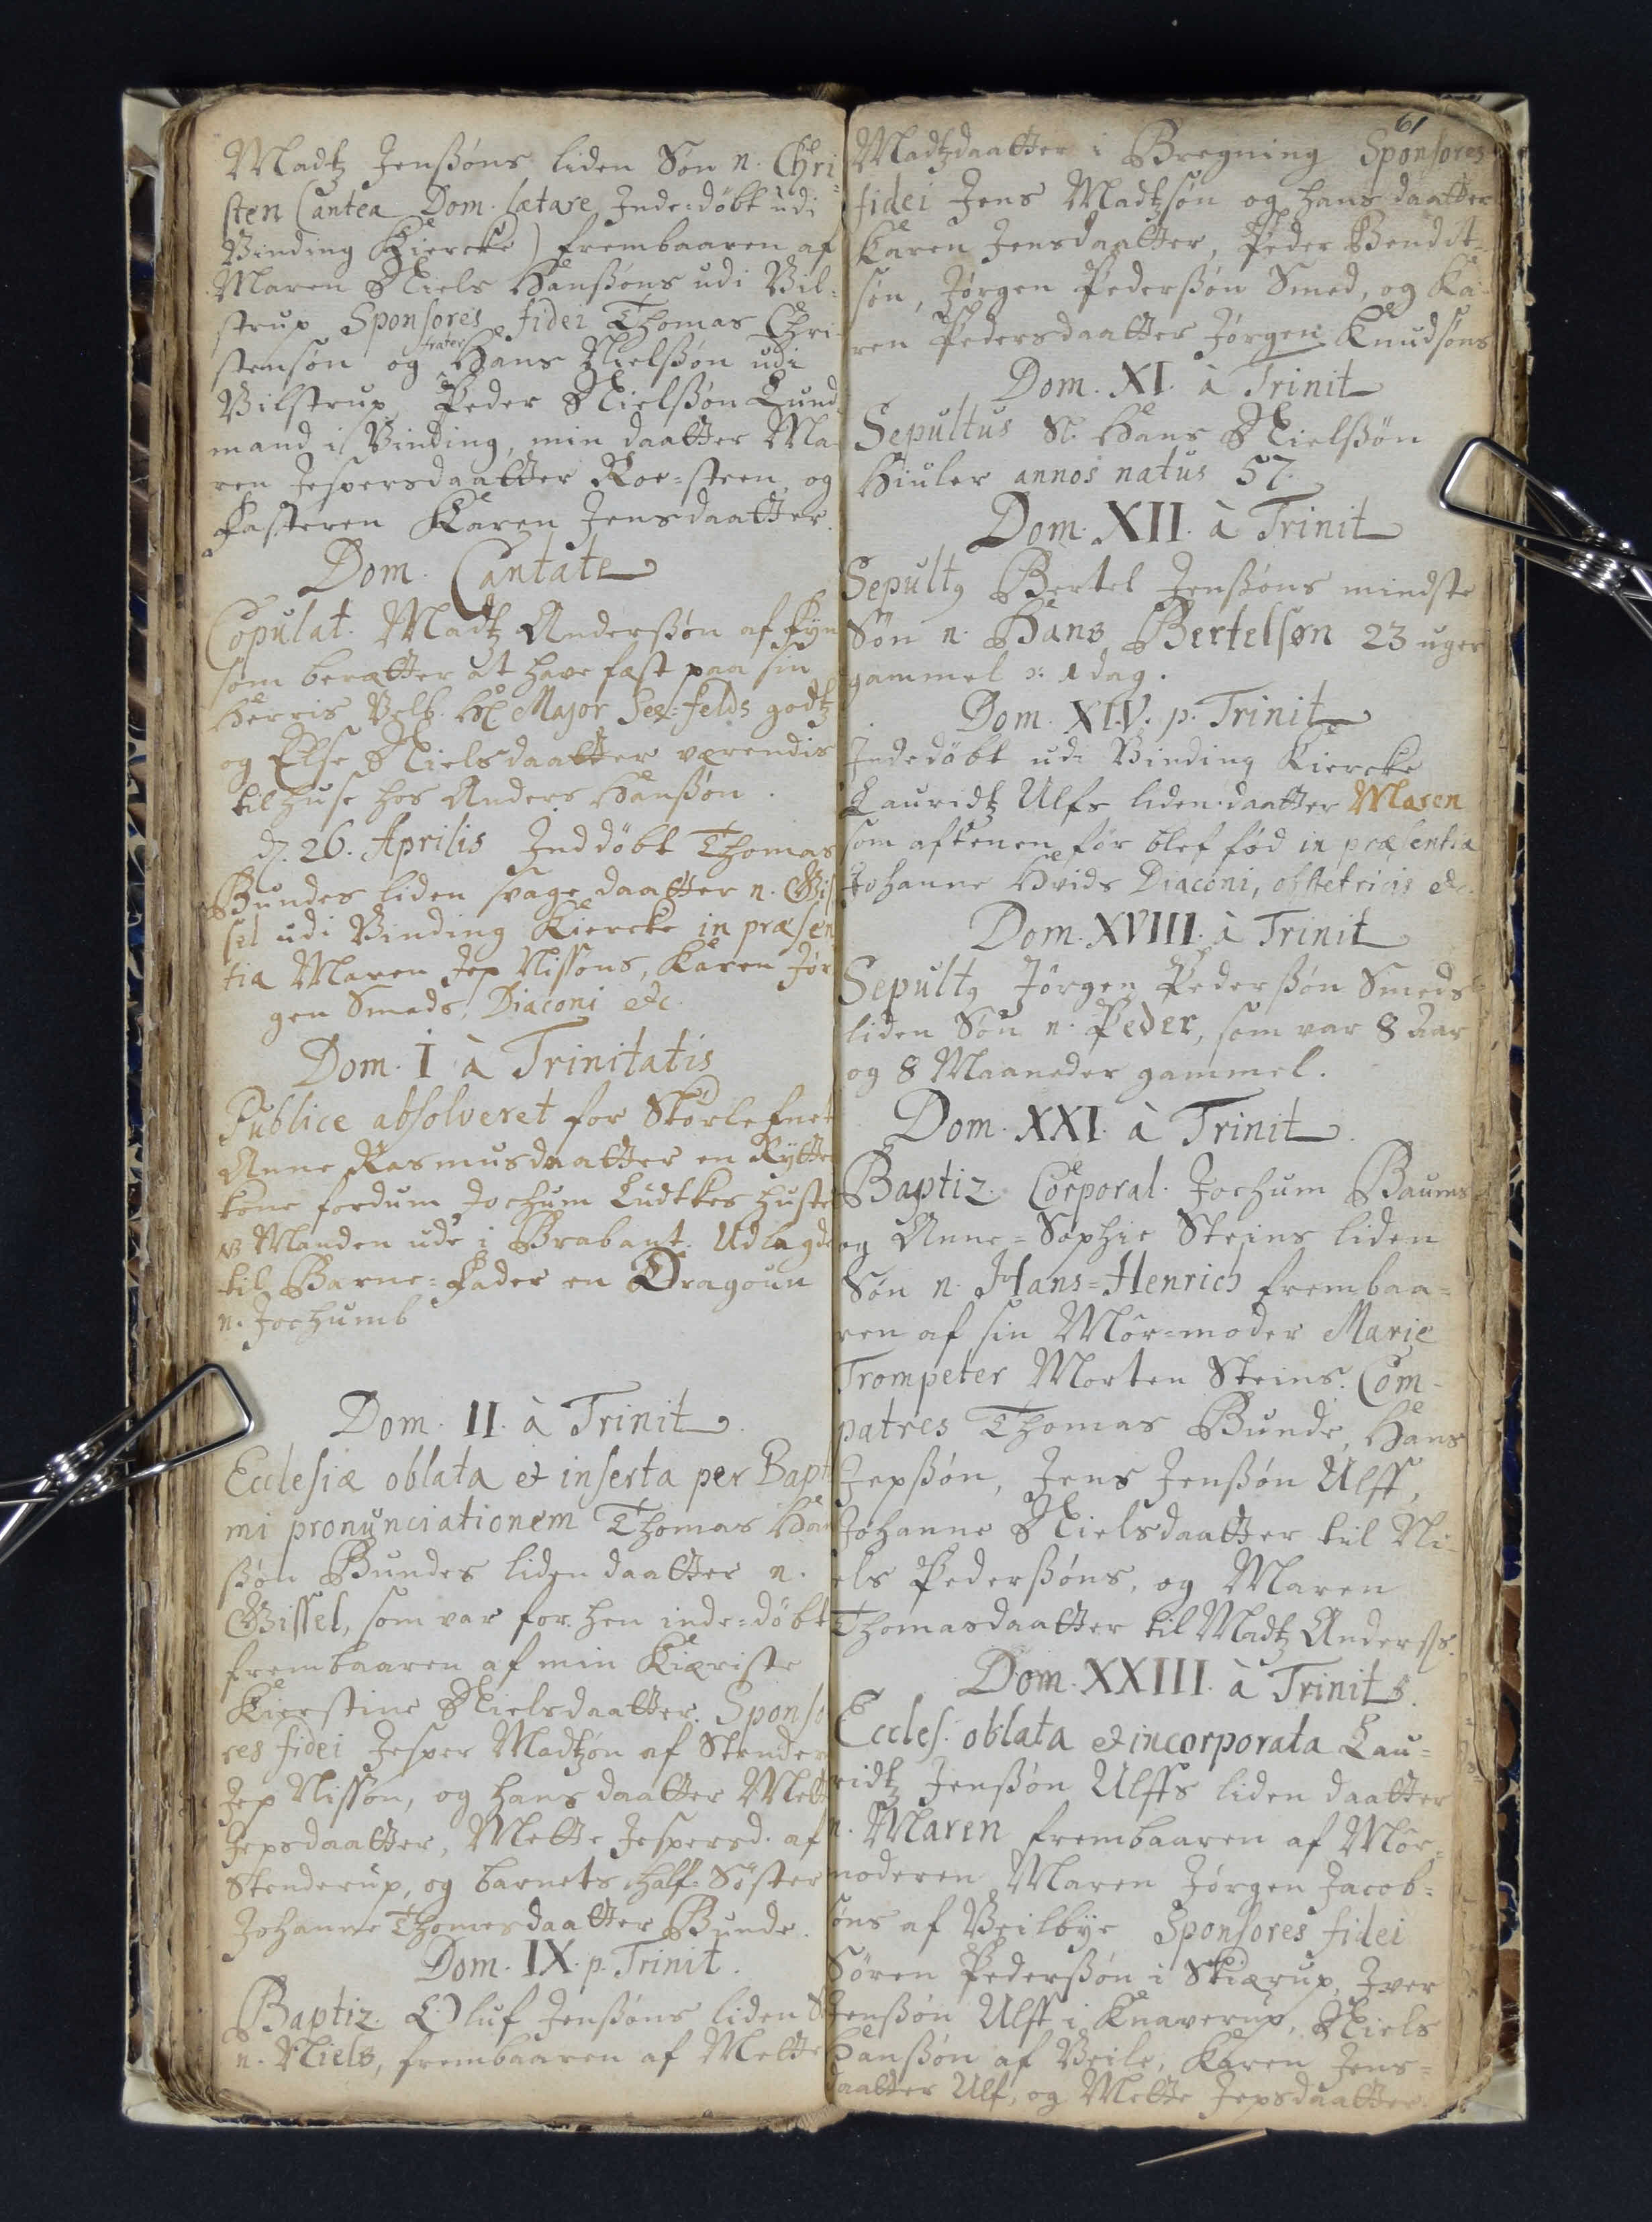Madtz Jensøns liden Søn n. Chri
sten (antea Dom. Lætare Inde=døbt udi
Vinding Kiercke) frembaaren af
Maren Niels Hanssøns udi Vil¬
strup Sponsores fidei Thomas Chri
frater##
stensøn og Hans Nielssøn udi
Vilstrup. Peder Nielssøn Lund
mand i Vinding, min daatter Ma
ren Jespersdaatter Roe=steen, og
Fasteren Karen Jensdaatter
Dom. Cantate
Copult. Madtz Anderssøn af Fyn
som berætter at have fæst paa sin 
Herris Velb. Hl. Major See=felds godtz
og Else Nielsdaatter værendis
til huse hos Anders Hanssøns
d. 26. Aprilis Inddøbt Thomas
Bundes liden svage daatter n. Gis
sel udi Vinding Kiercke in præsen
tia Maren Jep Nissøns, Karen Jør
gen Smeds. Diaconi etc
Dom. I a Trinitatis
Publice absolveret for Skørlefnet
Anne Rasmusdaatter en Rytter 
Kone fordum Jochum Ludtkes hustr 
NB. Manden ude i Brabant. Udlagde
til Barne=fader en Dragoun
n. Jochumb
Dom. II. a Trinit
Ecclesiæ oblata et inferta per Bapt 
mi pronunciationem Thomas Han
ssøn Bundes liden daatter n.
Gissel, som var for. hen inde=døbt,
frembaaren af min Kiæriste
Kierstine Nielsdaatter. Sponso
res fidei Jesper Madtzøn af Stender 
Jep Nissøn, og hans daatter Mette
Jepsdaatter, Mette Jeperssd. af
Stenderup, og barnets half=Søster
Johanne Thomasdaatter Bunde
Dom. IX. p. Trinit.
Baptiz. Oluf Jenssøns liden S 
n. Niels, frembaaren af Mette
61
Madtzdaatter i Breining Sponsores
fidei Jens Madtzsøn og hans daatter
Karen Jensdaatter, Peder Bendit¬
søn, Jørgen Pederssøn Smed, og Ka¬
ren Pedersdaatter Jørgen Knudsøns
Dom. XI. a Trinit
Sepultus Sl. Hans Nielssøn
Hiuler annos natus 57.
Dom. XII. a Trinit
Sepultq Bertel Jenssøns mindste
Søn n. Hans Bertelsøn 23 uger
gammel ↄ: 1 dag.
Dom. XI.V. p. Trinit
Indedøbt udi Vinding Kiercke
Lauridtz Ulffs liden daatter Maren
som aftenen før blef fød, in præsentia
Johanne Hvids, Diaconi, ob Actricis etc.
Dom. XVIII. a Trinit
Sepultq Jørgen Pederssøn Smeds
liden Søn n. Peder, som var 8 Aar
og 8 Maaneder gammel.
Dom. XXI. a Trinit
Baptiz. Corporal. Jochum Baums
og Anne=Sophie Steins liden
Søn n. Hans=Henrich frembaa¬
ren af sin Mor=moder Marie
Trompeter Morten Steins. Com¬
patres Thomas Bunde, Hans
Jepsøn', Jens Jenssøn Ulff
Johanne Nielsdaatter til Ni¬
els Pederssøns, og Maren
Thomasdaatter til Madtz Anderss.
Dom. XXIII. a Trinit
Eccles. oblata et incorporata Lau¬
ridtz Jenssøn Ulffs liden daatter
n. Maren, frembaaren af Mor¬
moderen Maren Jørgen Jacob¬
søns af Veilbye Sponsores fidei
Søren Pederssøn i Skiærup. Iver
Jensøn Ulff i Knaverup, Niels
Hanssøn af Veile, Karen Jens¬
daatter Ulf, og Mette Jepsdaatter.
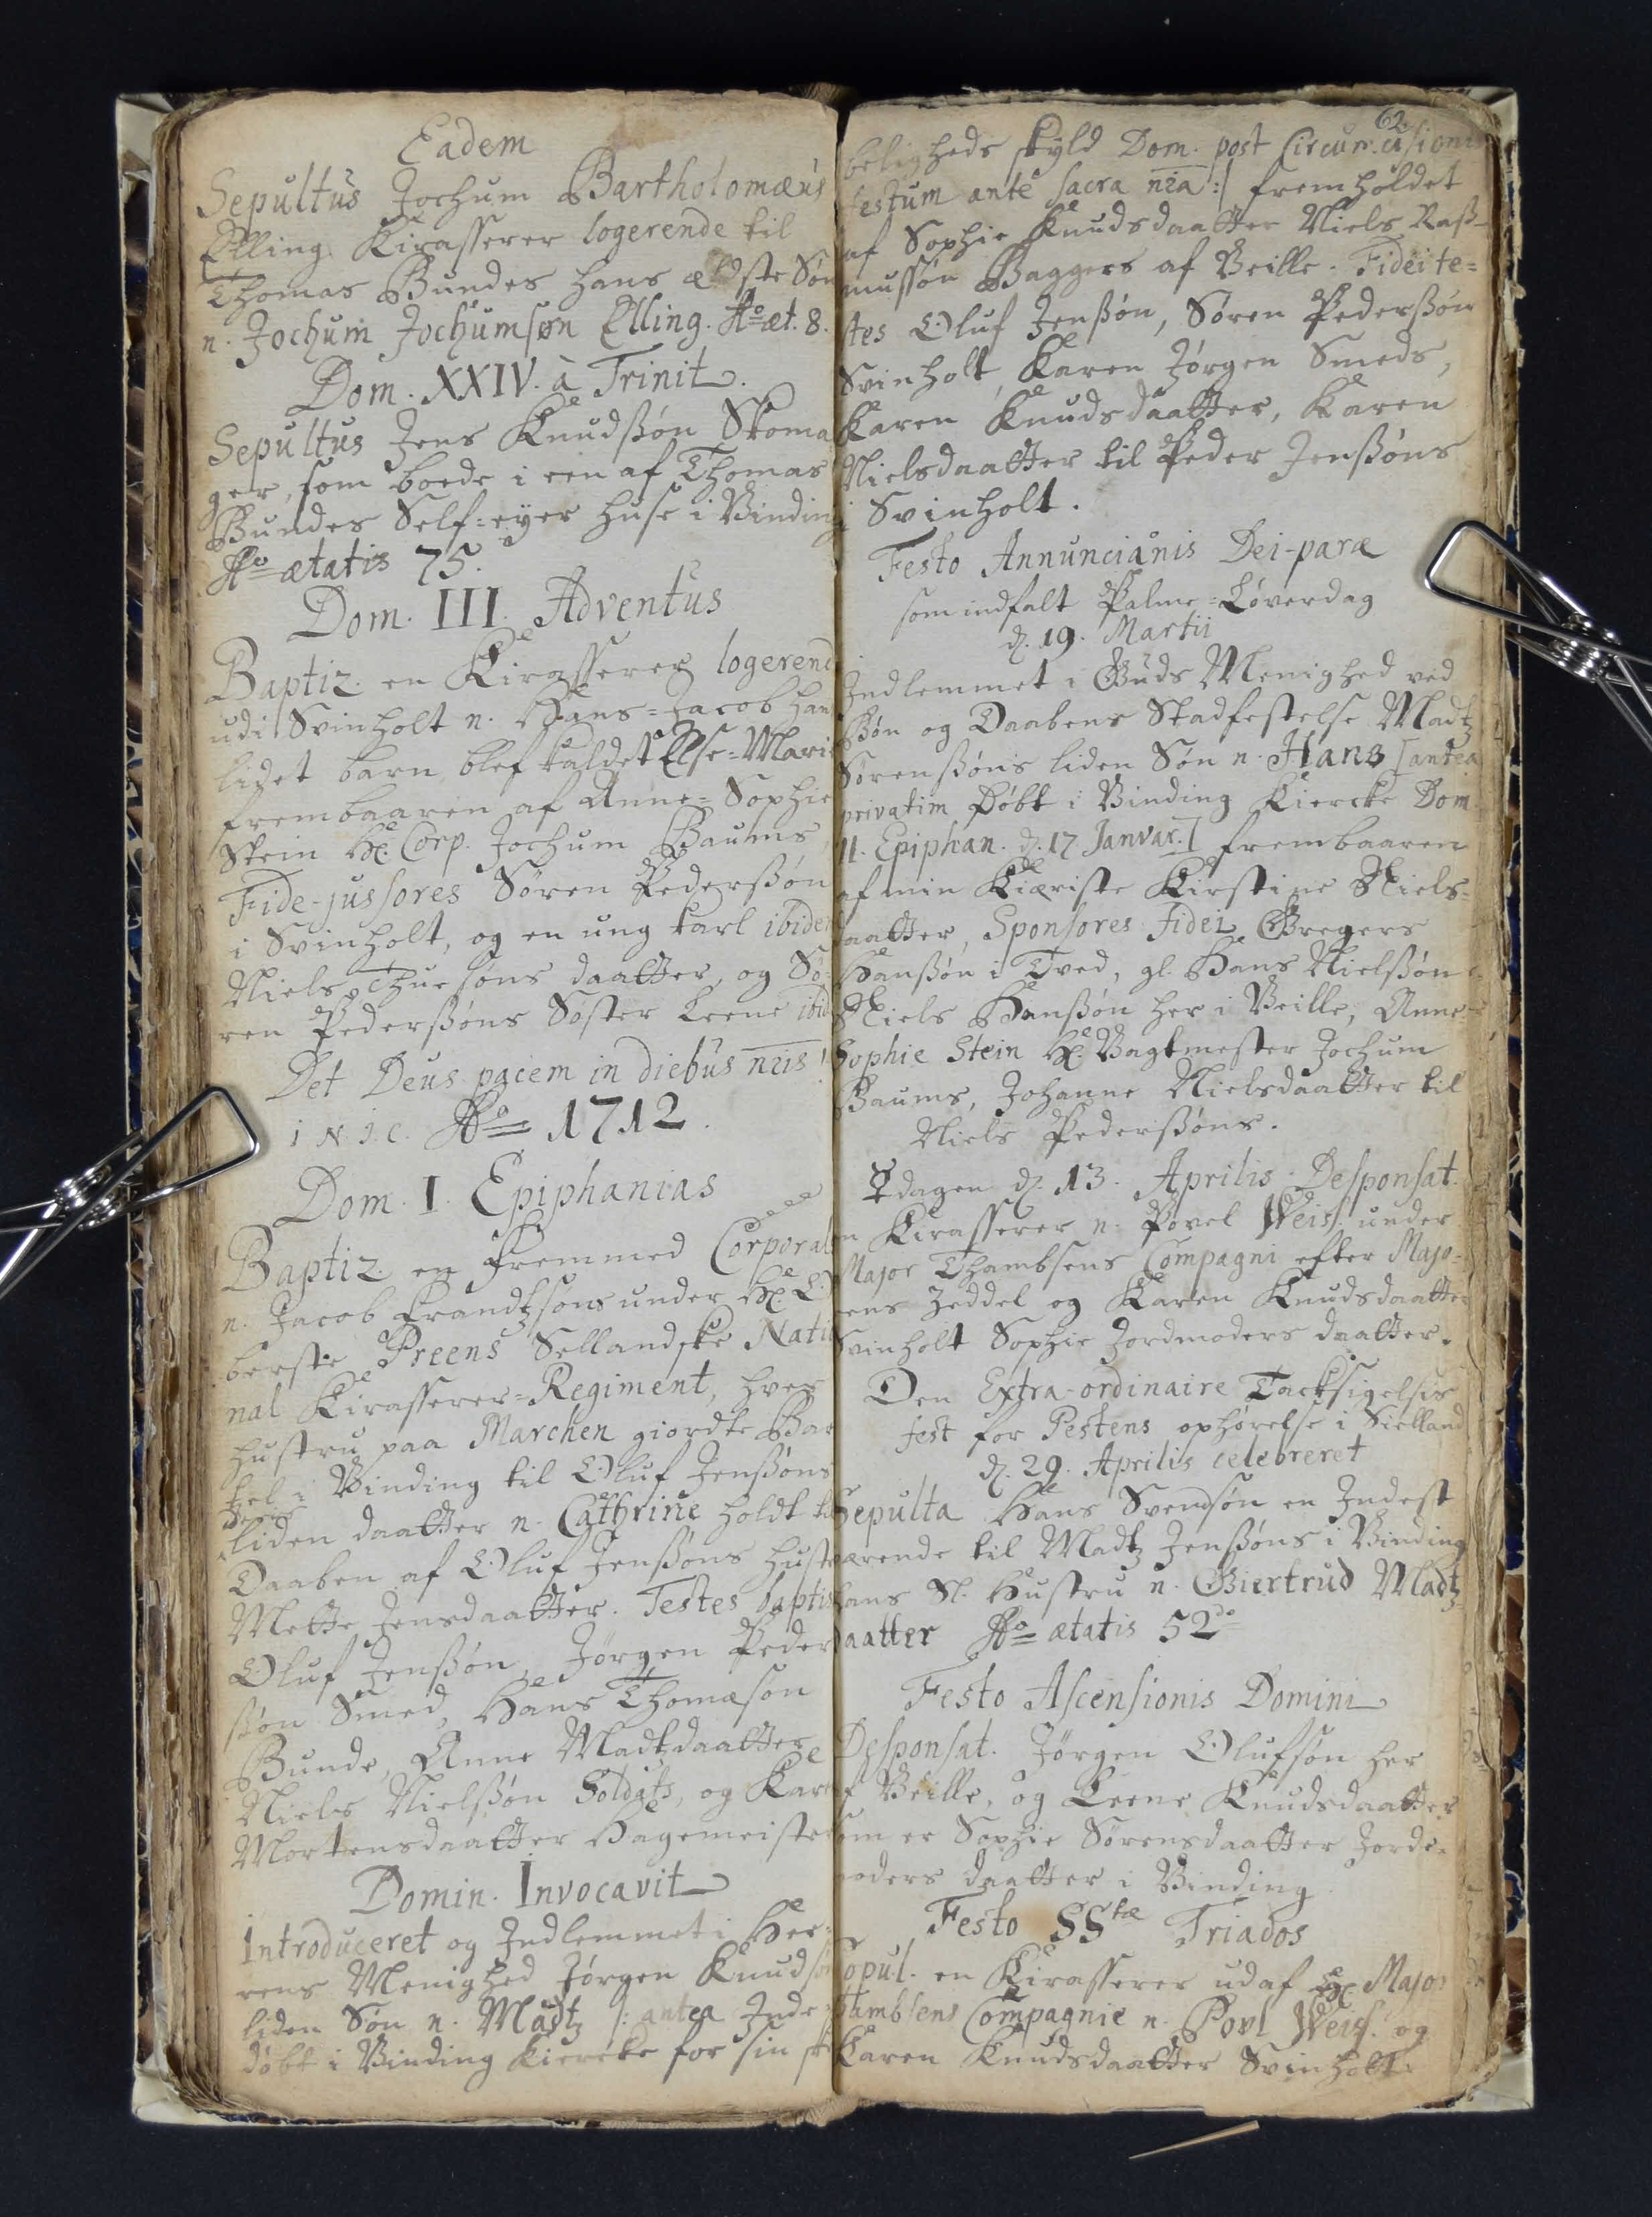Eadem
Sepultus Jochum Bartholomæus
Elling, Kirasserer logerende til
Thomas Bundes hans ældste Søn
n. Jochum Jochumsøn Elling. Ao æt. 8.
Dom. XXIV. a Trinit.
Sepultus Jens Knudssøn Skoma¬
ger, som boede i een af Thomas
Bundes Self=eyer huse i Vinding
Ao ætatis 75.
Dom. III. Adventus
Baptiz en Kirasserer logerend 
udi Svinholt n. Hans=Jacob han 
liden barn blef kaldet Else=Marie
frembaaren af Anne=Sophie
Stein Hl Corp. Jochum Baums
Fide-jussores Søren Pederssøn
i Svinholt, og en ung Karl ibide
Niels Thuesøns daatter, og Sø¬
ren Pederssøns Søster Leene ibid
Det Deus pacem in diebus n##s
I N J C Ao 1712
Dom. I Epiphanias
Baptiz en Fremmed Corporal 
n. Jacob Frandtzsøns under Hl. O
berste Preens Sellandske Nati 
nal Kirasserer=Regiment, hves
hustru paa Marchen giordte Bar
sel i Vinding til Oluf Jenssøns
deris
liden daatter n. Cathrine, holt til
Daaben af Oluf Jenssøns hustr 
Mette Jensdaatter. Testes baptis 
Oluf Jenssøn, Jørgen Peder
ssøn Smed, Hans Thomæsøn
Bunde, Anne Madtzdaatter
Niels Nielssøn Soldats, og Kare 
Mortensdaatter Hagemeister
Domin. Invocavit
Introduceret og Indlemmet i Her¬
rens Menighed Jørgen Knudsøn 
liden Søn n. Madtz /: antea Inde¬
døbt i Vinding Kiercke for sin skr 
62.
beligheds skyld Dom. post
festum ante sacra nia :/ fremholdet
af Sophie Knudsdaatter Niels Rass¬
mussøn Baggers af Veille. Fidei te¬
tes Oluf Jenssøn, Søren Pederssøn
Svinholt, Karen Jørgen Smeds
Karen Knudsdaatter, Karen
Nielsdaatter til Peder Jenssøns
i Svinholt.
Festo Annuncianis Dei-paræ
som indfalt Palme=Løverdag
d. 19 Martii
Indlemmet i Guds Menighed ved
Bøn og Daabens Stadfestelse Madtz
Sørenssøns liden Søn n. Hans [antea
privatim Døbt i Vinding Kiercke Dom.
II Epiphan. d. 17 Janvar.] frembaaren
af min Kiæriste Kierstine Niels¬
daatter, Sponsores fidei Gregers
Hanssøn i Tved. gl. Hans Nielssøn
Niels Hanssøn her i Veille, Anne
Sophie Stein Hl Vagtmester Jochum
Baums, Johanne Nielsdaatter til
Niels Pederssøns.
☿ dagen d. 13. Aprilis. Desponsat.
n Kirasserer n. Povel Weiss under
Major Thambsens Compagni efter Majo¬
rens Zeddel og Karen Knudsdaatter
Svinholt Sophie Jordmoders daatter.
Den Extra-ordinaire Tacksigelsis
Fest for Pestens ophørelse i Sielland
d. 29. Aprilis celebreret
Sepulta Hans Svendsøn en Inderst
ærende til Madtz Jenssøns i Vinding
hans Sl. Hustru n. Giertrud Madtz¬
aatter Ao ætatis 52do
Festo Ascensionis Domini
Desponsat Jørgen Olufsøn her
f Veille, og Leene Knudsdaatter
som er Sophie Sørensdaatter Jorde¬
oders daatter i Vinding
Festo SStæ Triados
Copul. en Kirasserer udaf ## Major
ambsens Compagnie n. Povl Weiss og
Karen Knudsdaatter Svinholt.